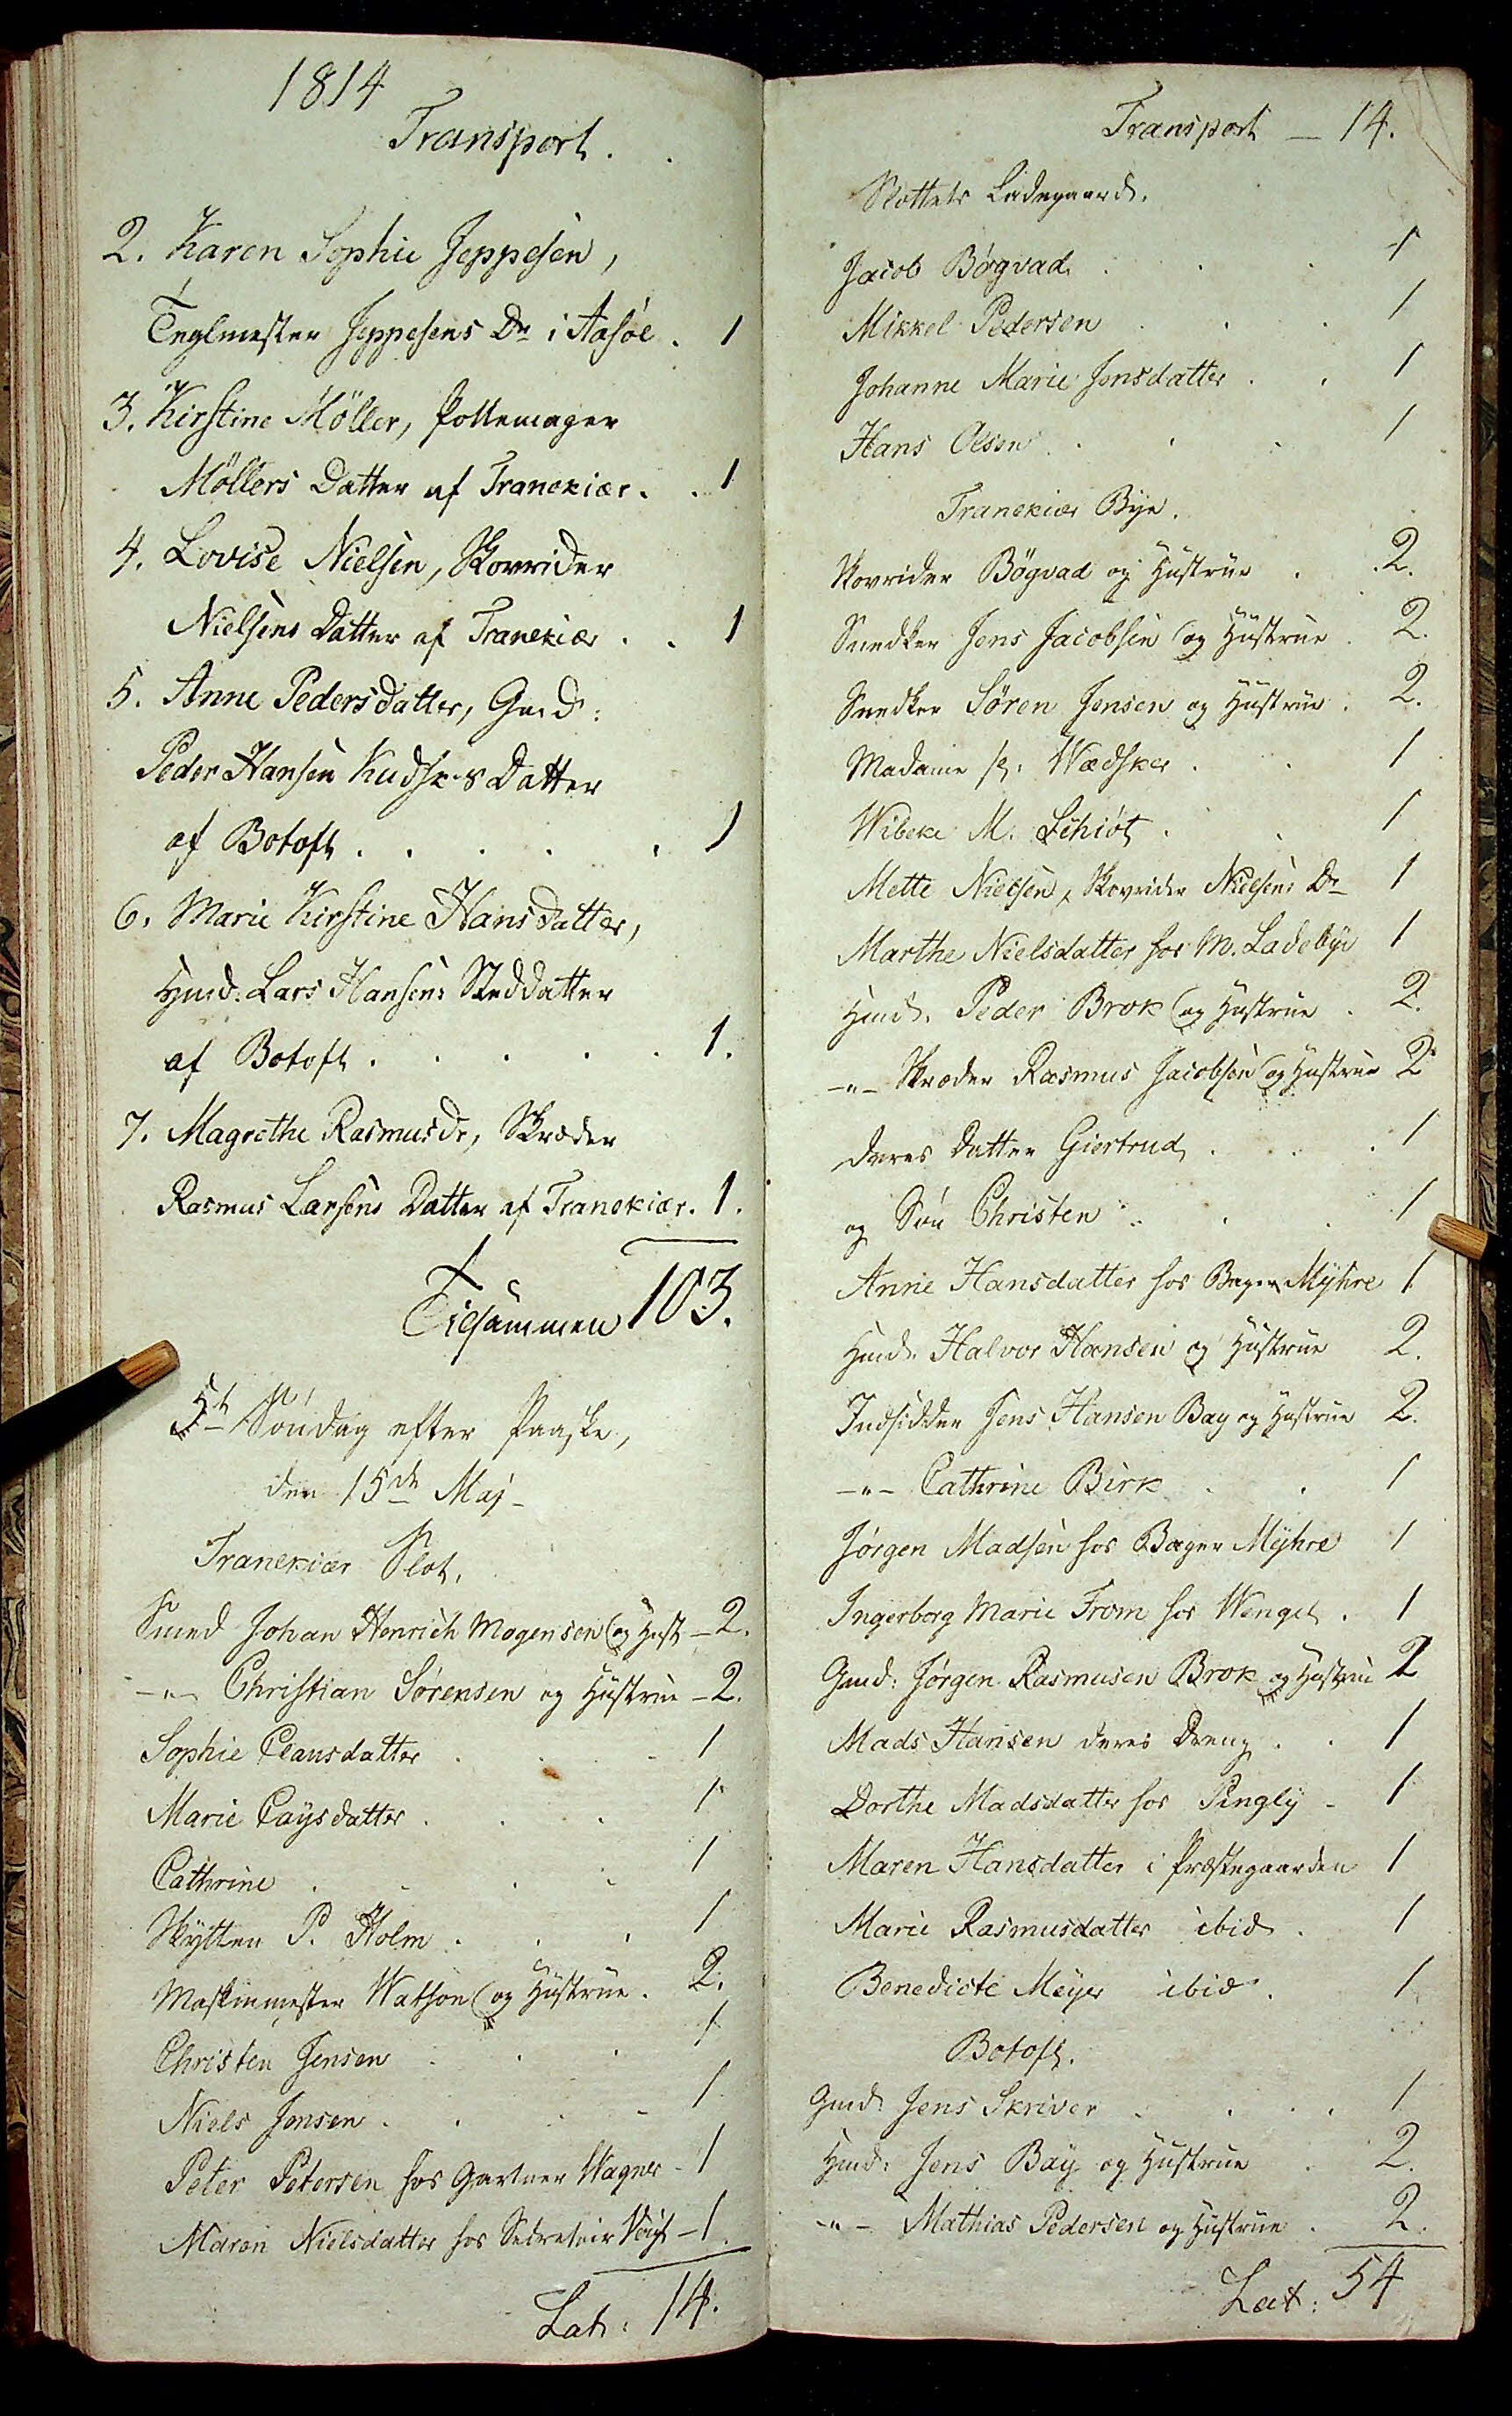1814
Transport . .
2. Karen Sophie Jeppesen,
Teglmester Jeppesens Dr i Aasøe . 1
3. Kirstine Møller, Pottemager
Møllers Datter af Tranekiær . . 1
4. Lovise Nielsen, Skovrider
Nielsens Datter af Tranekiær . . 1
5. Anne Pedersdatter, Gmd:
Peder Hansen Kudskes Datter
af Botoft . . . . . 1
6. Marie Kirstine Hansdatter,
Hmd: Lars Hansen Steddatter
af Botoft . . . . . 1
7. Magrethe Rasmusdr, Skræder
Rasmus Larsens Datter af Tranekiær . 1.
Tilsammen 103.
5te Søndag efter Paaske,
den 15de Maj -
Tranekiær Slot.
Smed Johan Henrich Mogensens og Hustr - 2
-"- Christian Sørensen og Hustrue - 2.
Sophie Clausdatter . . . . 1
Marie Caysdatter . . . . 1
Catrine . . . . 1
Skytten P Holm . . . 1
Maskinmester Watson og Hustrue . 2.
Christen Jensen . . . 1
Niels Jensen . . . . 1
Pater Petersen hos Gartner Wagner . 1
Maren Nielsdatter hos Setretair Voigt - 1
Lat: 14.
Transport - 14.
Slottets Ladegaard.
Jacob Bøgvad . . . 1
Mikkel Pedersen . . . 1
Johanne Marie Jensdatter . . 1
Hans Olsen . . . . 1
Tranekiær Bye.
Skovrider Bøgvad og Hustrue . . 2.
Snedker Jens Jacobsen og Hustrue . 2.
Snedker Søren Jensen og Hustrue . 2.
Madam sl: Wædsker . . 1
Wibeke M: Schiøt . . 1
Mette Nielsen, Skovrider Nielsens Dr 1
Marthe Nielsdatter hos M: Ladebye 1
Hmd Peder Brok og Hustrue . 2.
-"- Skræder Rasmus Jacobsen og Hustrue 2
deres Datter Giertrud . . . 1
og Søn Christen . . . 1
Anne Hansdatter hos Bager Myhre 1
Hmd Halvor Hansen og Hustrue 2.
Indsidder Jens Hansen Bay og Hustrue 2.
-"- Cathrine Birk . . 1
Jørgen Madsen hos Bager Myhre 1
Ingeborg Marie From for Wengel . 1
Gmd: Jørgen Rasmusen Brok og Hustru 2
Mads Hansen deres Dreng . . 1
Dorthe Madsdatter hos Pingly -1
Maren Hansdatter i Præstegaarden 1
Marie Rasmusdatter ibid . 1
Bendicte Meyer ibid . 1
Botoft.
Gmd: Jens Skriver . . . . 1
Hmd: Jens Bay og Hustrue . 2.
-"- Mathias Pedersen og Hustrue . 2.
Lat: 54
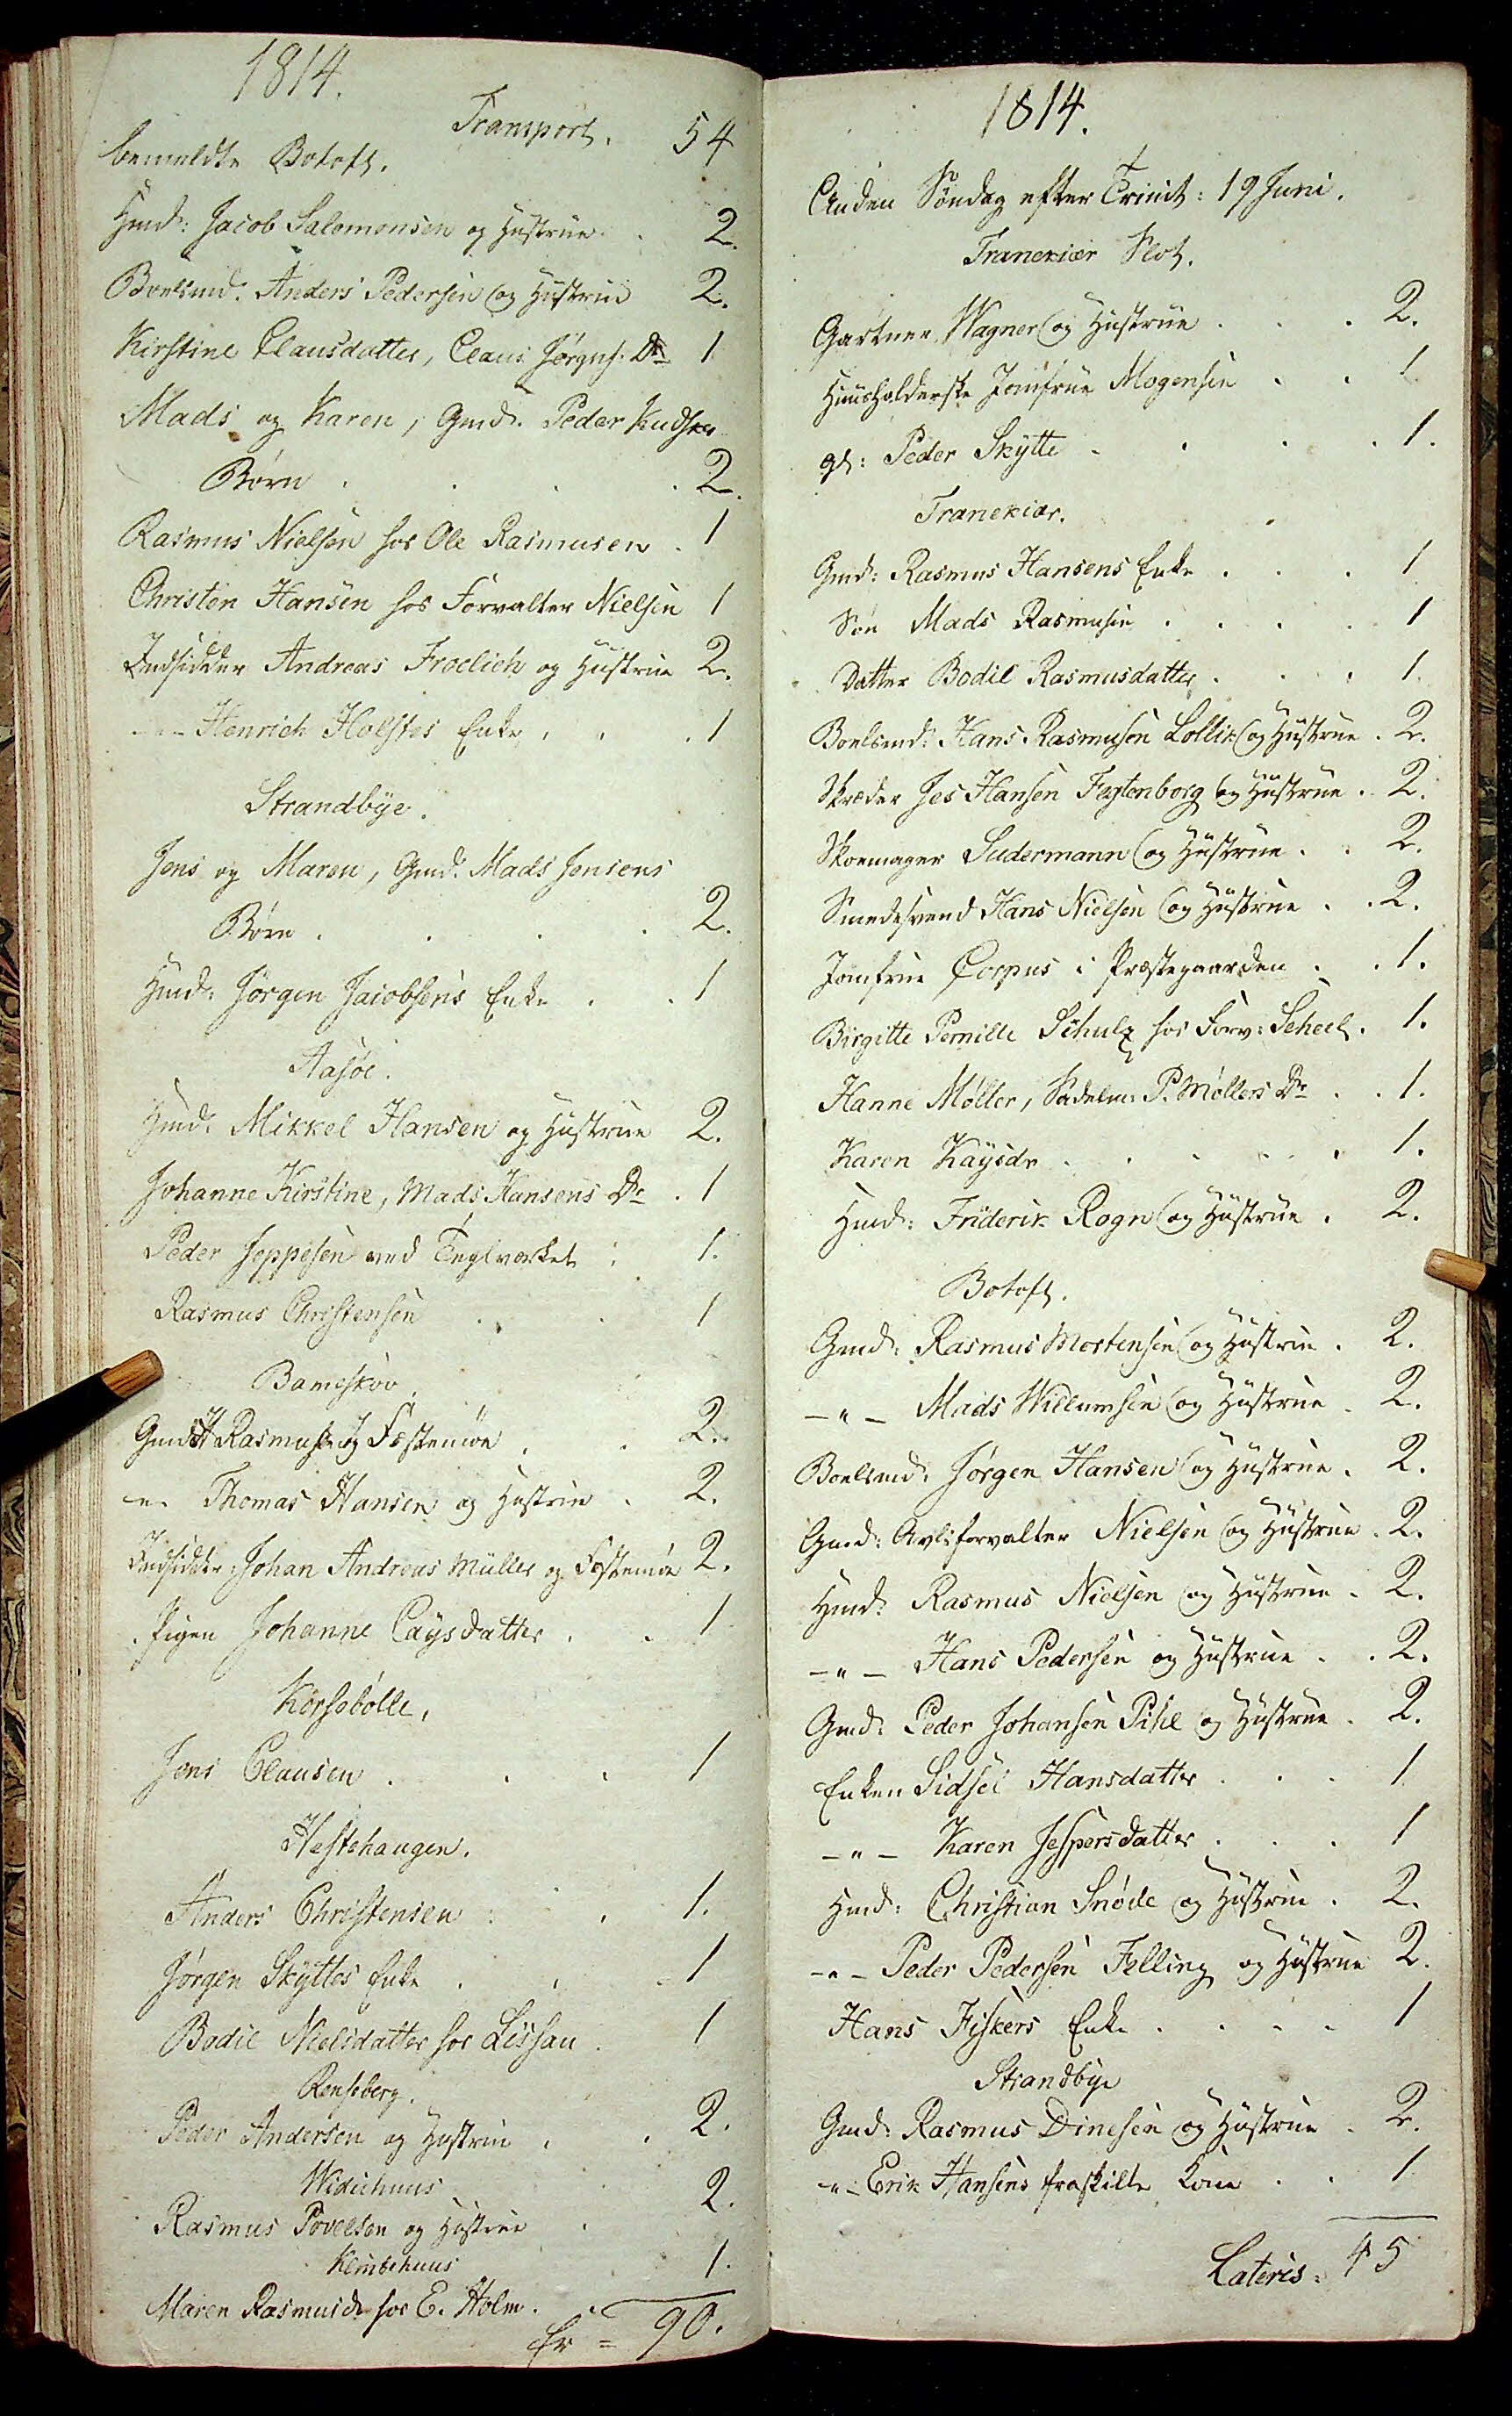1814.
Transport. 54
bemeldte Botoft.
Hmd: Jacob Salomonsen og Hustrue . 2.
Boelsmd. Anders Pedersen og Hustrue 2.
Kirstine Clausdatter, Claus Jørgens. Dr 1
Mads og Karen, Gm.d Peder Kudskes
Børn . . . . 2.
Rasmus Nielsen hos Ole Rasmusen . 1
Christen Hansen hos Forvalter Nielsen 1
Indsidder Andreas Froclich og Hustrue 2.
-"- Henrich Holstes Enke . . . 1
Stranbye.
Jens og Maren, Gmd. Mads Jensens
Børn . . . . 2.
Hmd: Jørgen Jacobsens Enke . . 1
Aasøe.
Hmd. Mikkel Hansen og Hustrue 2.
Johanne Kirstine, Mads Hansens Dr . 1
Peder Jeppesen ved Teglværket : 1.
Rasmus Christensen . . 1
Bameskov
Gmd H Rasmusen og Fæstemøe . . 2.
-"- Thomas Hansen og Hustrue . 2.
Indsidder: Johan Andreas Muller og Fæstemøe 2.
Pigen Johanne Caysdatter . . 1
Korsebølle.
Jens Clausen . . . 1
Hestehaugen.
Anders Christensen : . 1.
Jørgen Skyttes Enke . . . 1
Bodil Nielsdatter hos Lissau . 1
Renseberg
Peder Andersen og Hustrue . . 2.
Widiehuus
Rasmus Povelsen og Hustrue . 2.
Klintehuus
Maren Rasmusdr hos E. Holm . . 1.
Er = 90
1814.
Anden Søndag efter Trinit: 19 Juni.
Tranekiær Slot.
Gartner Wagner og Hustrue . . .  2.
Huusholderske Jomfrue Mogensen . . 1.
gl: Peder Skytte . . . . 1.
Tranekiær.
Gmd: Rasmus Hansens Enke . . . 1
Søn Mads Rasmusen . . . . 1
Datter Bodil Rasmusdatter . . . 1.
Boelsmd: Hans Rasmusen Lollik og Hustrue . 2.
Skræder Jes Hansen Fegtenborg og Hustrue . 2.
Skoemager Sudermann og Hustrue . . 2.
Smedesved Hans Nielsen og Hustrue . . 2.
Jomfrue Corpus i Præstegaarden . . 1.
Birgitte Pernille Schulz hos Forv: Scheel . 1.
Hanne Møller, Saddelm: P. Møllers Dr . . 1.
Karen Kaysdr . . . . . . 1.
Hmd: Friderik Rogn og Hustrue . 2.
Botoft.
Gmd: Rasmus Mortensen og Hustrue . 2.
-"- Mads Willumsen og Hustrue . 2.
Boelsmd: Jørgen Hansen og Hustrue . 2.
Gmd: Avlsforvalter Nielsen og Hustrue . 2.
Hmd: Rasmus Nielsen og Hustrue . 2.
-"- Hans Pedersen og Hustrue . . 2.
Gmd: Peder Johansen Pihl og Hustrue .2.
Enken Sidsel Hansdatter . . . 1.
-"- Maren Jespersdatter . . . 1
Hmd: Christian Snøde og Hustrue . 2
-"- Peder Pedersen Felling og Hustrue 2.
Hans Fiskers Enke . . . . 1-
Strandbye
Gmd: Rasmus Dinesen og Hustrue . 2.
-"- Erik Hansens fraskilte Kone . . 1
Lateris : 45